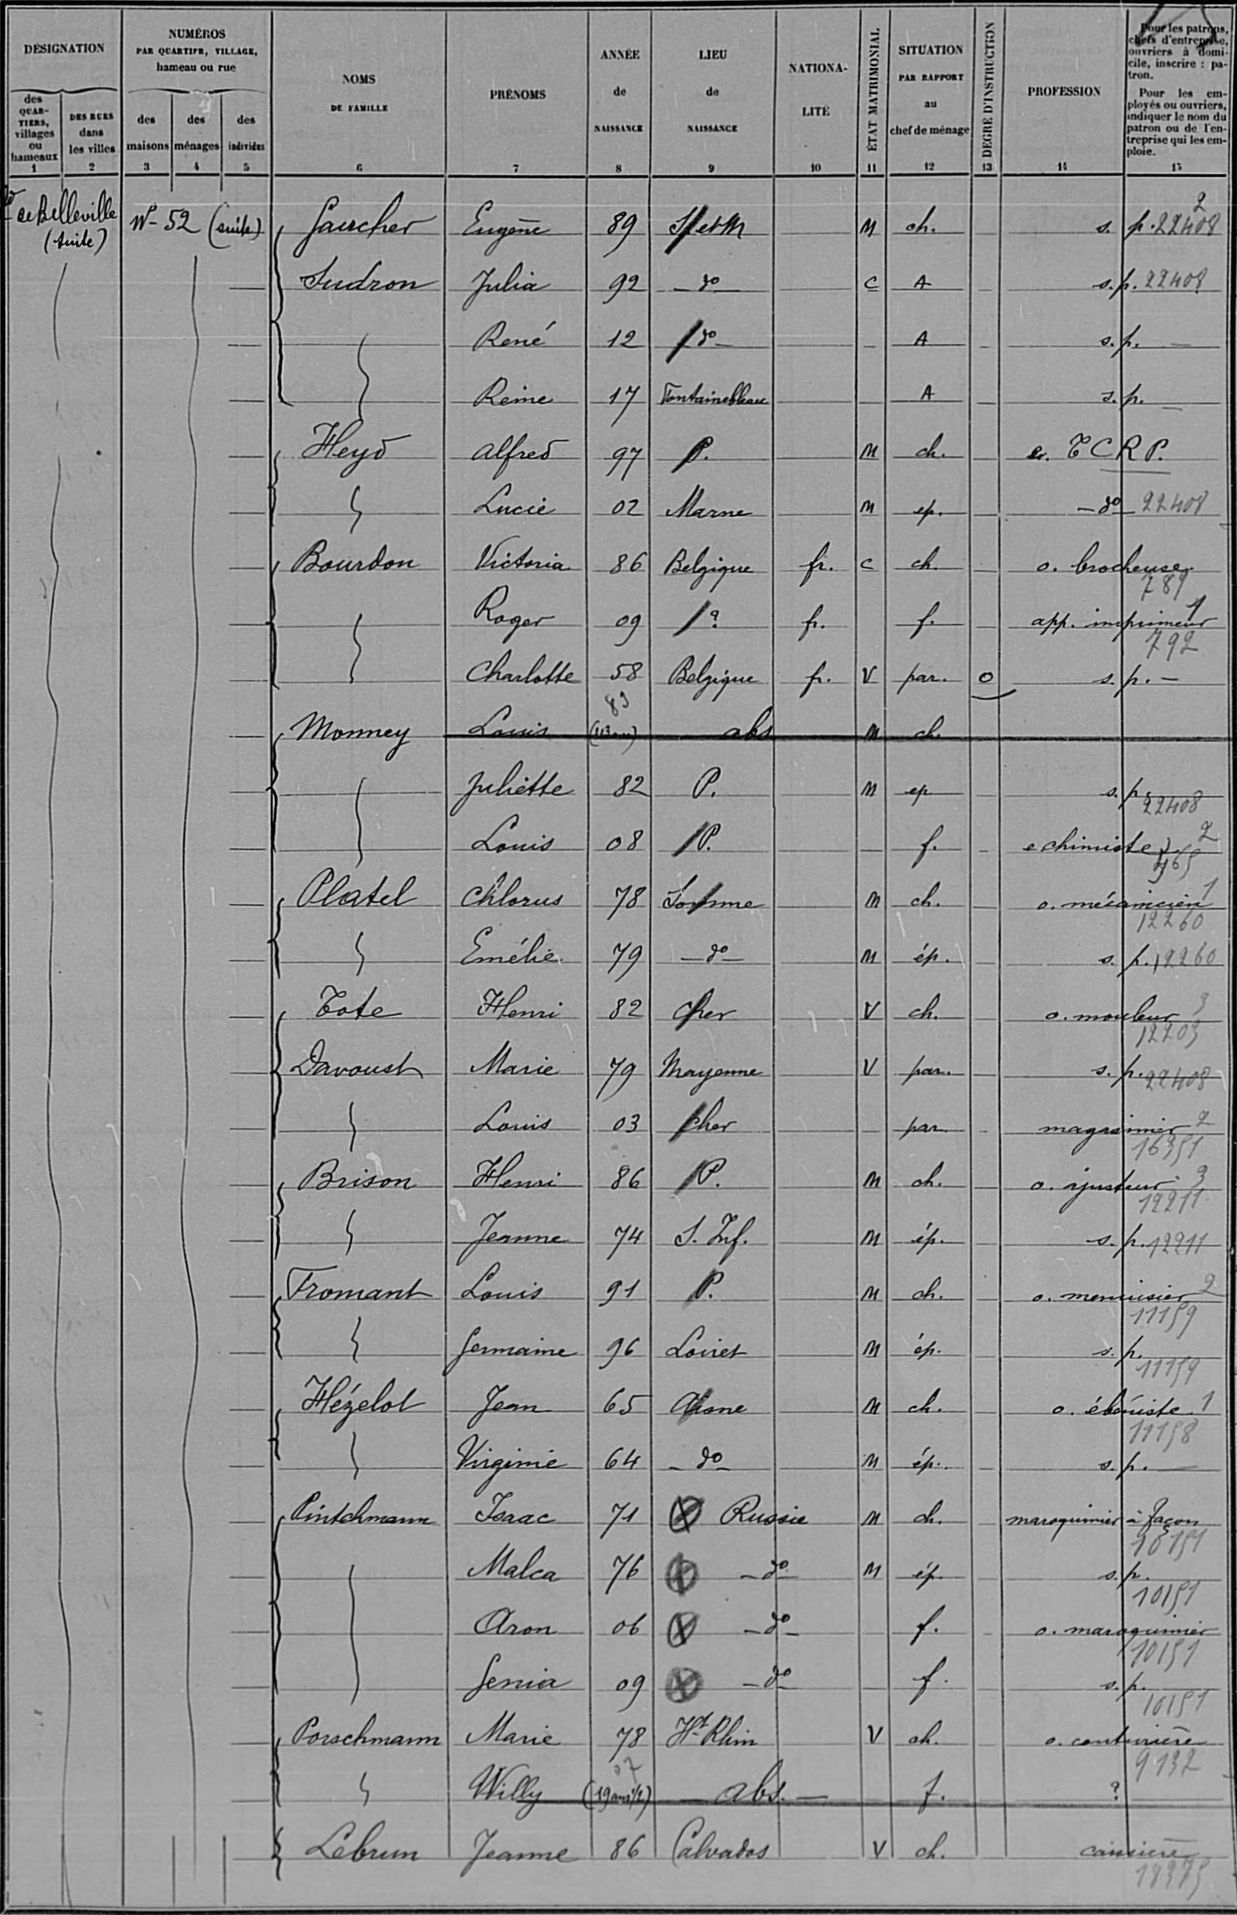Gaucher/Eugène/89/S et M/¤/M/ch /¤/s p /224082
Sudron/Julia/92/d°/¤/C/A/¤/s p /22408
¤/René/12/d°/¤/¤/A/¤/s p /¤/¤
Reine/17/Fontainebleau/¤/¤/A/¤/s p /¤/¤
Heyd/Alfred/97/P /¤/M/ch /¤/e C CRP /¤/¤
¤/Lucie/02/Marne/¤/M/ep /¤/d°/22408
Bourdon/Victoria/86/Belgique/fr /C/ch /¤/o brocheuse/¤
¤/Roger/09/¤/fr /¤/f /¤/app imprimeur/¤
¤/Charlotte/58/Belgique/fr /V/par /o/s p /¤/¤
Monney/Louis/(43 ans) 83/abs/¤/M/ch /¤/¤/¤/¤
¤/Juliette/82/P /¤/M/ep/¤/s p /¤
¤/Louis/08/P /¤/¤/f /¤/e chimiste/¤
Platel/Chlorus/78/Somme/¤/M/ch /¤/o mécanicien/¤
¤/Emélie/79/d°/¤/M/ép /¤/s p /12260
Cote/Henri/82/Cher/¤/V/ch /¤/o mouleur/¤
Davoust/Marie/79/Mayenne/¤/V/par /¤/s p /22408
¤/Louis/03/Cher/¤/¤/par/¤/magasinier/¤
Brison/Henri/86/P /¤/M/ch /¤/o ajusteur/¤
¤/Jeanne/74/S Inf /¤/M/ép /¤/s p /12211
Fromant/Louis/91/P /¤/M/ch /¤/o menuisier/¤
¤/Germaine/96/Loiret/¤/M/ép /¤/s p /¤
Hégelot/Jean/65/Aisne/¤/M/ch /¤/o ébéniste/¤
¤/Virginie/64/d°/¤/M/ép /¤/s p /¤
Pinschmann/Isaac/71/Russie/¤/M/ch /¤/maroquinier à façon/¤
¤/Malca/76/d°/¤/M/ép /¤/s p /¤
¤/Aron/06/d°/¤/¤/f /¤/o maroquinier/¤
¤/Genia/09/d°/¤/¤/f /¤/s p /¤
Porschmann/Marie/78/Ht Rhin/¤/V/ch /¤/o couturière/¤
¤/Willy/(19 ans 1 2) 07/abs /¤/¤/f /¤/¤/¤/¤
Lebrun/Jeanne/86/Calvados/¤/V/ch /¤/caissière/¤